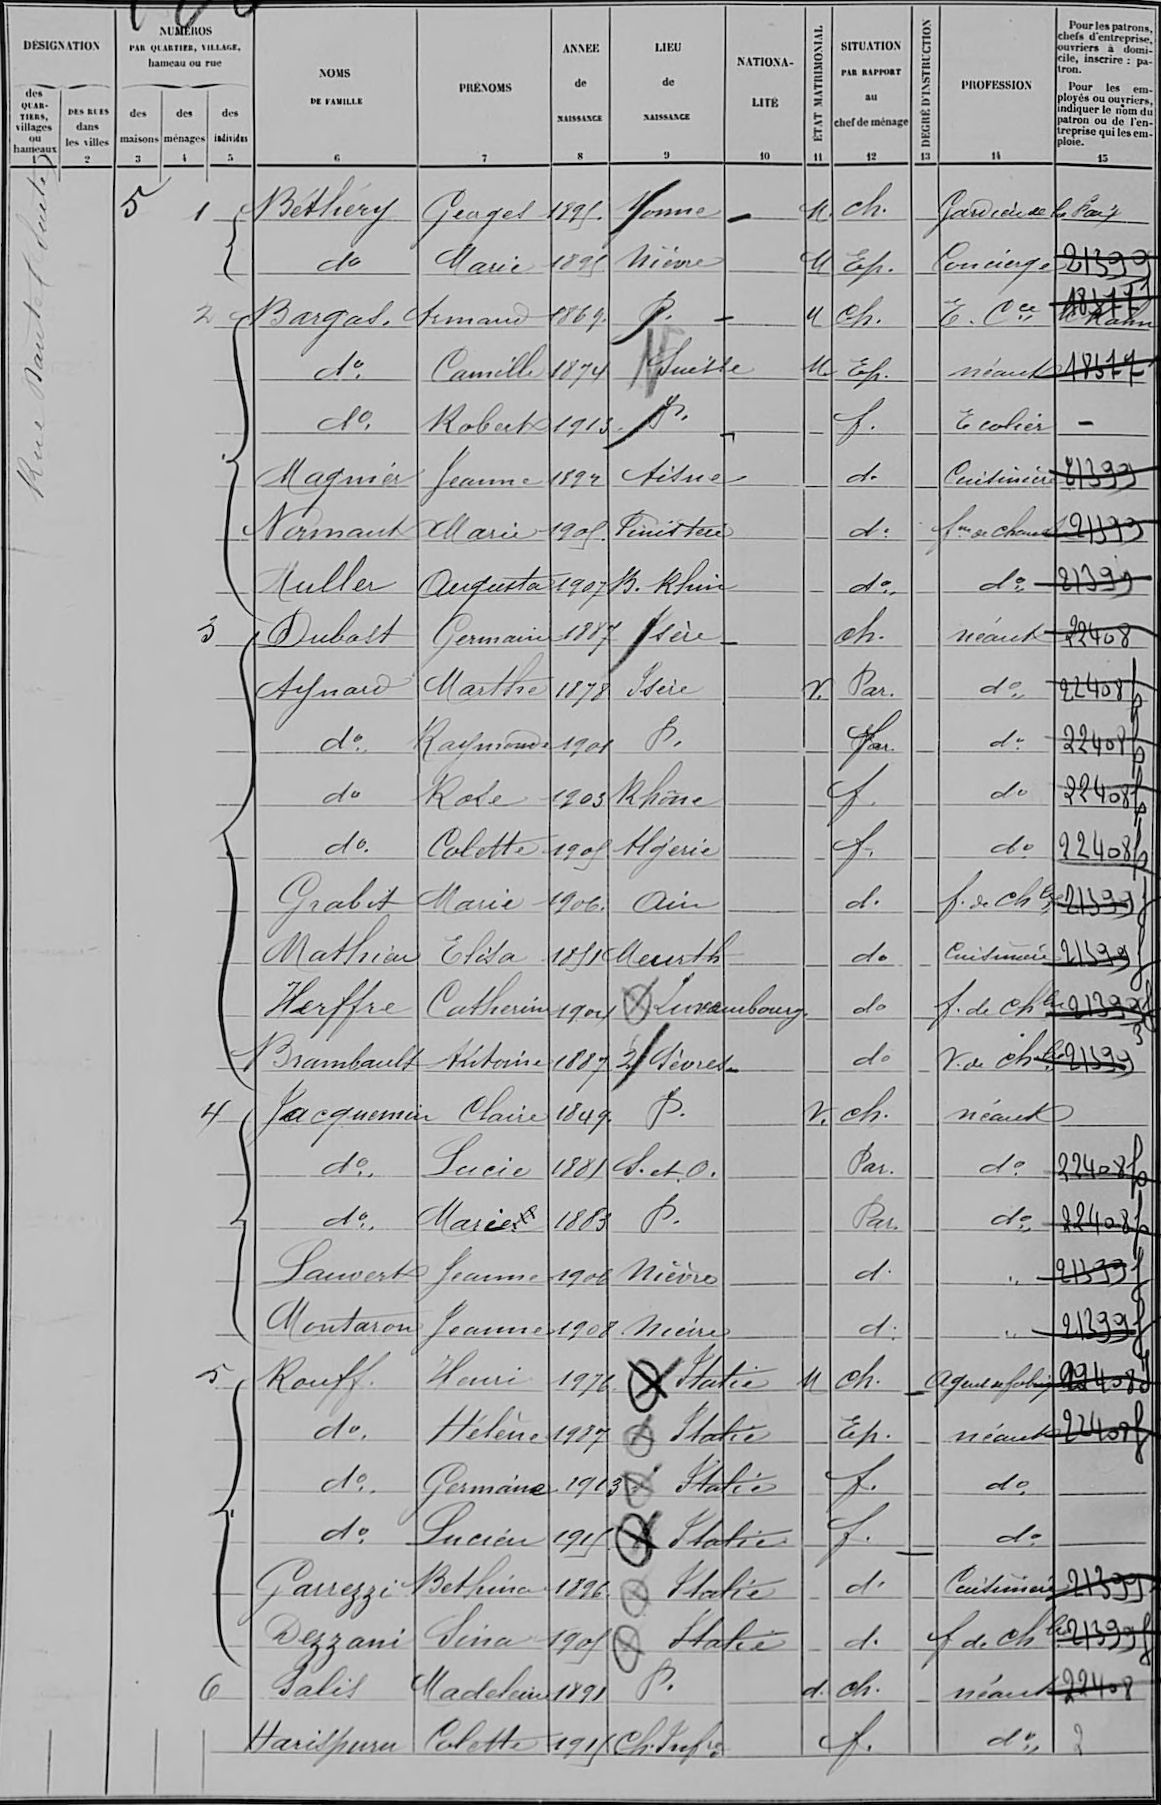Béthéry/Georges/1895/Yonne/¤/M/Ch /¤/Gardien de la Paix/¤
d°/Marie/1895/Nièvre/¤/M/Ep /¤/Concierge/21399
Bargas/Armand/1869/P /¤/M/Ch /¤/E Cce/Ve Kahn?18377
d°/Camille/1874/Suisse/¤/M/Ep /¤/néant/18377
d°/Robert/1913/P /¤/¤/f /¤/Ecolier/¤
Magnier/Jeanne/1894/Aisne/¤/¤/d /¤/Cuisinière/21399
Vermant/Marie/1905/Finistère/¤/¤/d /¤/fme de chambre/21399
Muller/Augusta/1907/B Rhin/¤/¤/d°/¤/d°/21399
Dubost/Germain/1887/Isère/¤/¤/ch /¤/néant/22408
Aynard/MArthe/1878/Isère/¤/V /Par /¤/d°/22408 f
d°/Raymonde/1901/P /¤/¤/Par /¤/d°/22408 f
d°/Rose/1903/Rhône/¤/¤/f /¤/d°/22408 f
d°/Colette/1905/Algérie/¤/¤/f /¤/d°/22408 f
Grabit/Marie/1906/Ain/¤/¤/d /¤/f de chbre/21399 f
Mathieu/Elisa/1851/Meurth/¤/¤/d /¤/Cuisinière/21399 f
Herffre/Catherine/1904/Luxembourg/¤/¤/d /¤/f de chbre/21399 f
Brambault/Antoine/1887/2 Sèvres/¤/¤/d /¤/V de chbre/21399
Jacquemin/Claire/1849/P /¤/V /ch /¤/néant/¤
d°/Lucie/1881/S et O /¤/¤/Par /¤/d°/22408 f
d°/Marie/1883/P /¤/¤/Par/¤/d°/22408 f
Lauvert/Jeanne/1906/Nièvre/¤/¤/d /¤/¤/21399 f
Montaron/Jeanne/1908/Nièvre/¤/¤/d /¤/¤/21399 f
Rouff/Henri/1976/Italie/¤/M/ch /¤/Agent de fabrique/22408 J
d°/Hélène/1987/Italie/¤/¤/Ep /¤/néant/22408 f
d°/Germaine/1913/Italie/¤/¤/f /¤/d°/¤
d°/Lucien/1915/Italie/¤/¤/f /¤/d°/¤
Garrezi/Bathina/1896/Italie/¤/¤/d /¤/Cuisinière/21399
Dezzani/Sina/1905/Italie/¤/¤/d /¤/f de chbre/21399 f
Salis/Madeleine/1891/P /¤/d/ch /¤/néant/22408
Harispuru/Colette/1915/Ch Infre/¤/¤/f /¤/d°/2
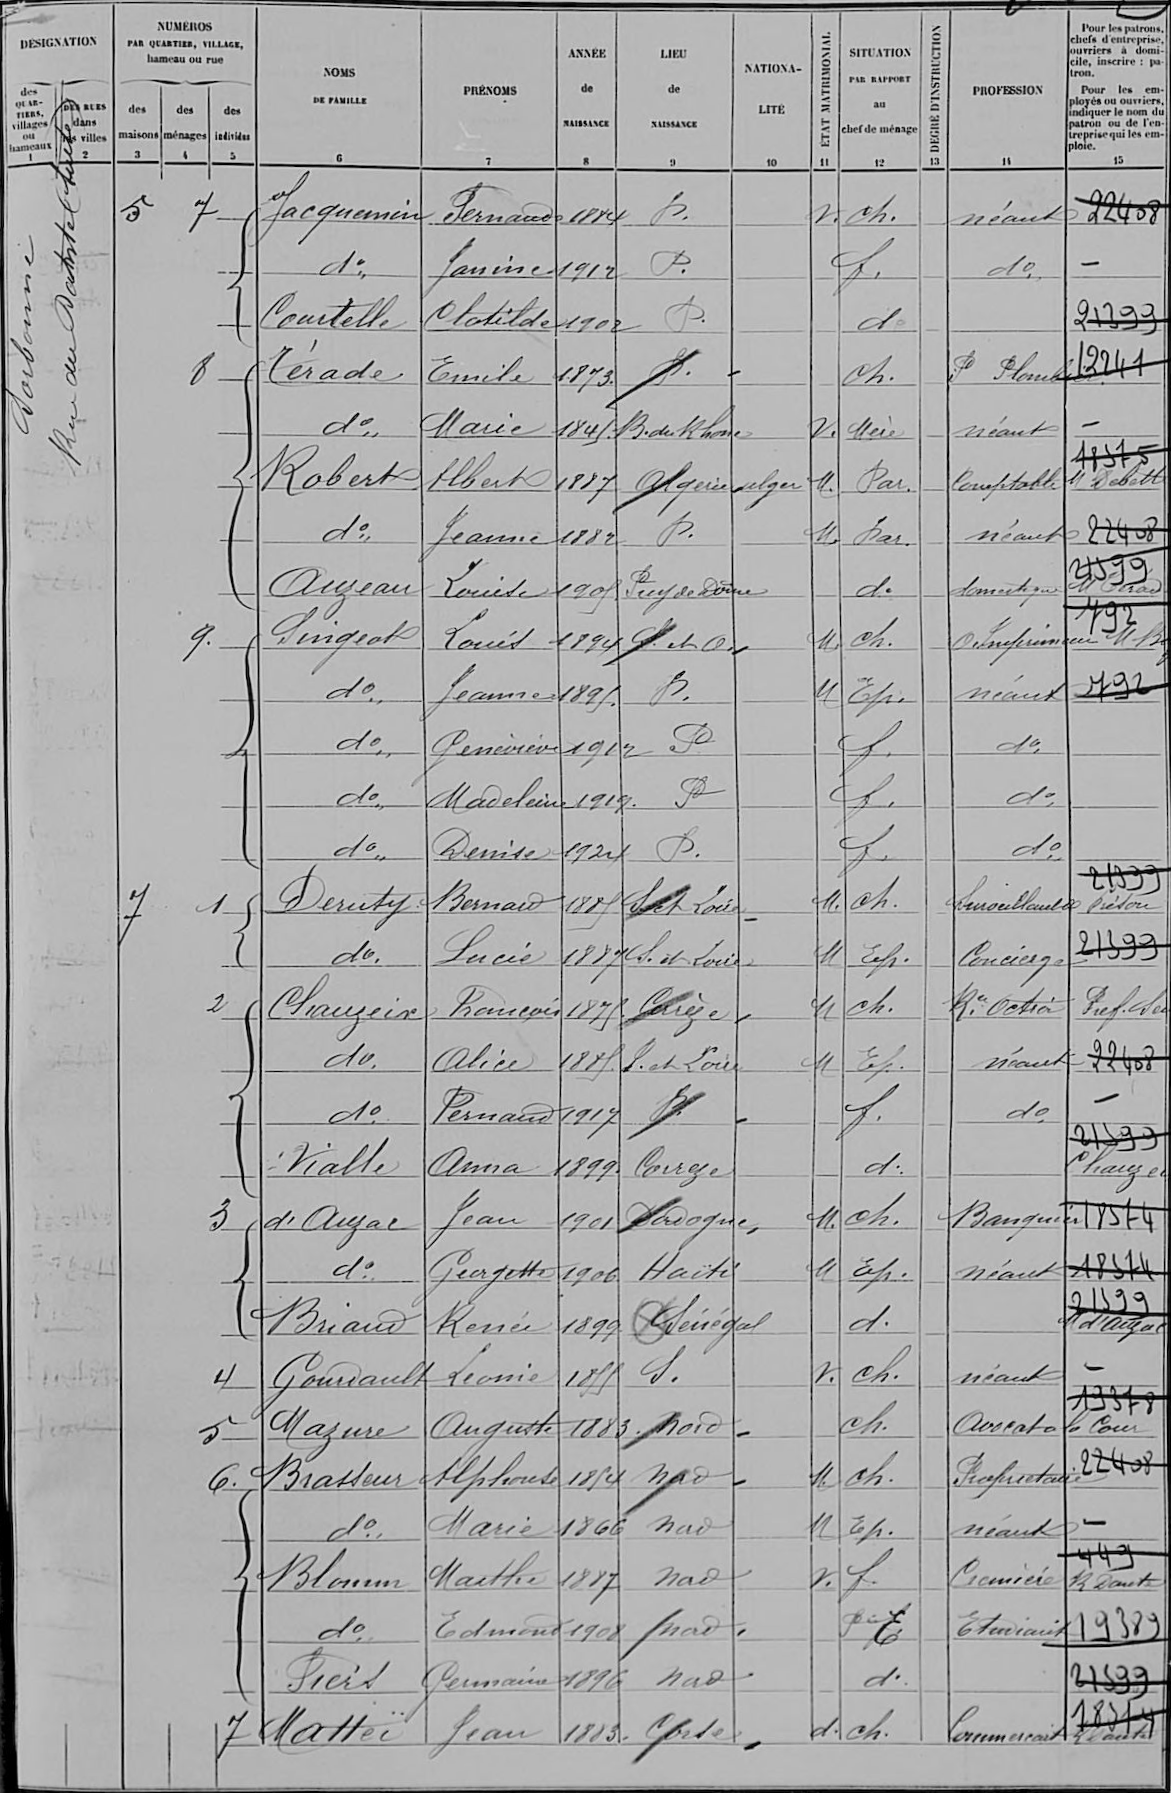Jacquemin/Fernando/1884/P /¤/V /Ch /¤/néant/22408
d°/Janine/1912/P /¤/¤/f /¤/d°/¤
Courtelle/Clotidle/1902/P /¤/¤/d/¤/¤/21399
Térade/Emile/1873/P /¤/¤/ch /¤/P Plombier/12241
d°/Marie/1845/B du Rhhone/¤/V /Mère/¤/néant/¤
Robert/Albert/1887/Algérie/Alger/M/Par /¤/Comptavle/M Debett?18375
d°/Jeanne/1882/P /¤/M/Par /¤/néant/22408
Auzeau/Louise/1905/Puy de Dôme/¤/¤/d /¤/domestique/M Térard?21399
Singeot/Louis/1894/S et O /¤/M/ch /¤/O Imprimeur/M Be?792
d°/Jeanne/1895/P /¤/M/Ep /¤/néant/792
d°/Geneviève/1912/P/¤/¤/f /¤/d°/¤
d°/Madeleine/1919/P/¤/¤/f /¤/d°/¤
d°/Denise/1924/P /¤/¤/f /¤/d°/¤
Deruty/Bernard/1885/S et Loire/¤/M /Ch /¤/Surveillant de Prison/?21399
d°/Lucie/1887/S et Loire/¤/M/Ep /¤/Concierge/21399
Chauzeix/François/1875/Corrèze/¤/M/ch /¤/Rce Octroi/Pref Sei
d°/Alice/1885/I et Loire/¤/M/Ep /¤/néant/22408
d°/Fernand/1917/P /¤/¤/f /¤/d°/¤
Vialle/Anna/1899/Corrèze/¤/¤/d /¤/¤/Chauzeix?2399
d'Auzac/Jean/1901/Dordogne/¤/M /ch /¤/Banquier/18374
d°/Georgette/1906/Haïti/¤/M/Ep /¤/néant/18374
Briaud/Renée/1899/Sénégal/¤/¤/d /¤/¤/M D'Auzac?21399
Gourrault/léonie/1855/S /¤/V /Ch /¤/néant/¤
Mazure/Auguste/1883/nord/¤/¤/ch /¤/Avocat à la cour/?19378
Brasseur/Alphonse/1854/nord/¤/M /Ch /¤/Propriétaire/22408
d°/Marie/1866/Nord/¤/M/Ep /¤/néant/¤
Blonnin/Marthe/1887/Nord/¤/V /f /¤/Cremière/R Dante?449
d°/Edmond/1908/Nord/¤/¤/P E /¤/Etudiant/19389
Siers/Germaine/1896/Nord/¤/¤/d /¤/¤/21399
Matteï/Jean/1883/Corse/¤/d/ch /¤/Commerçant/R Dante?18374